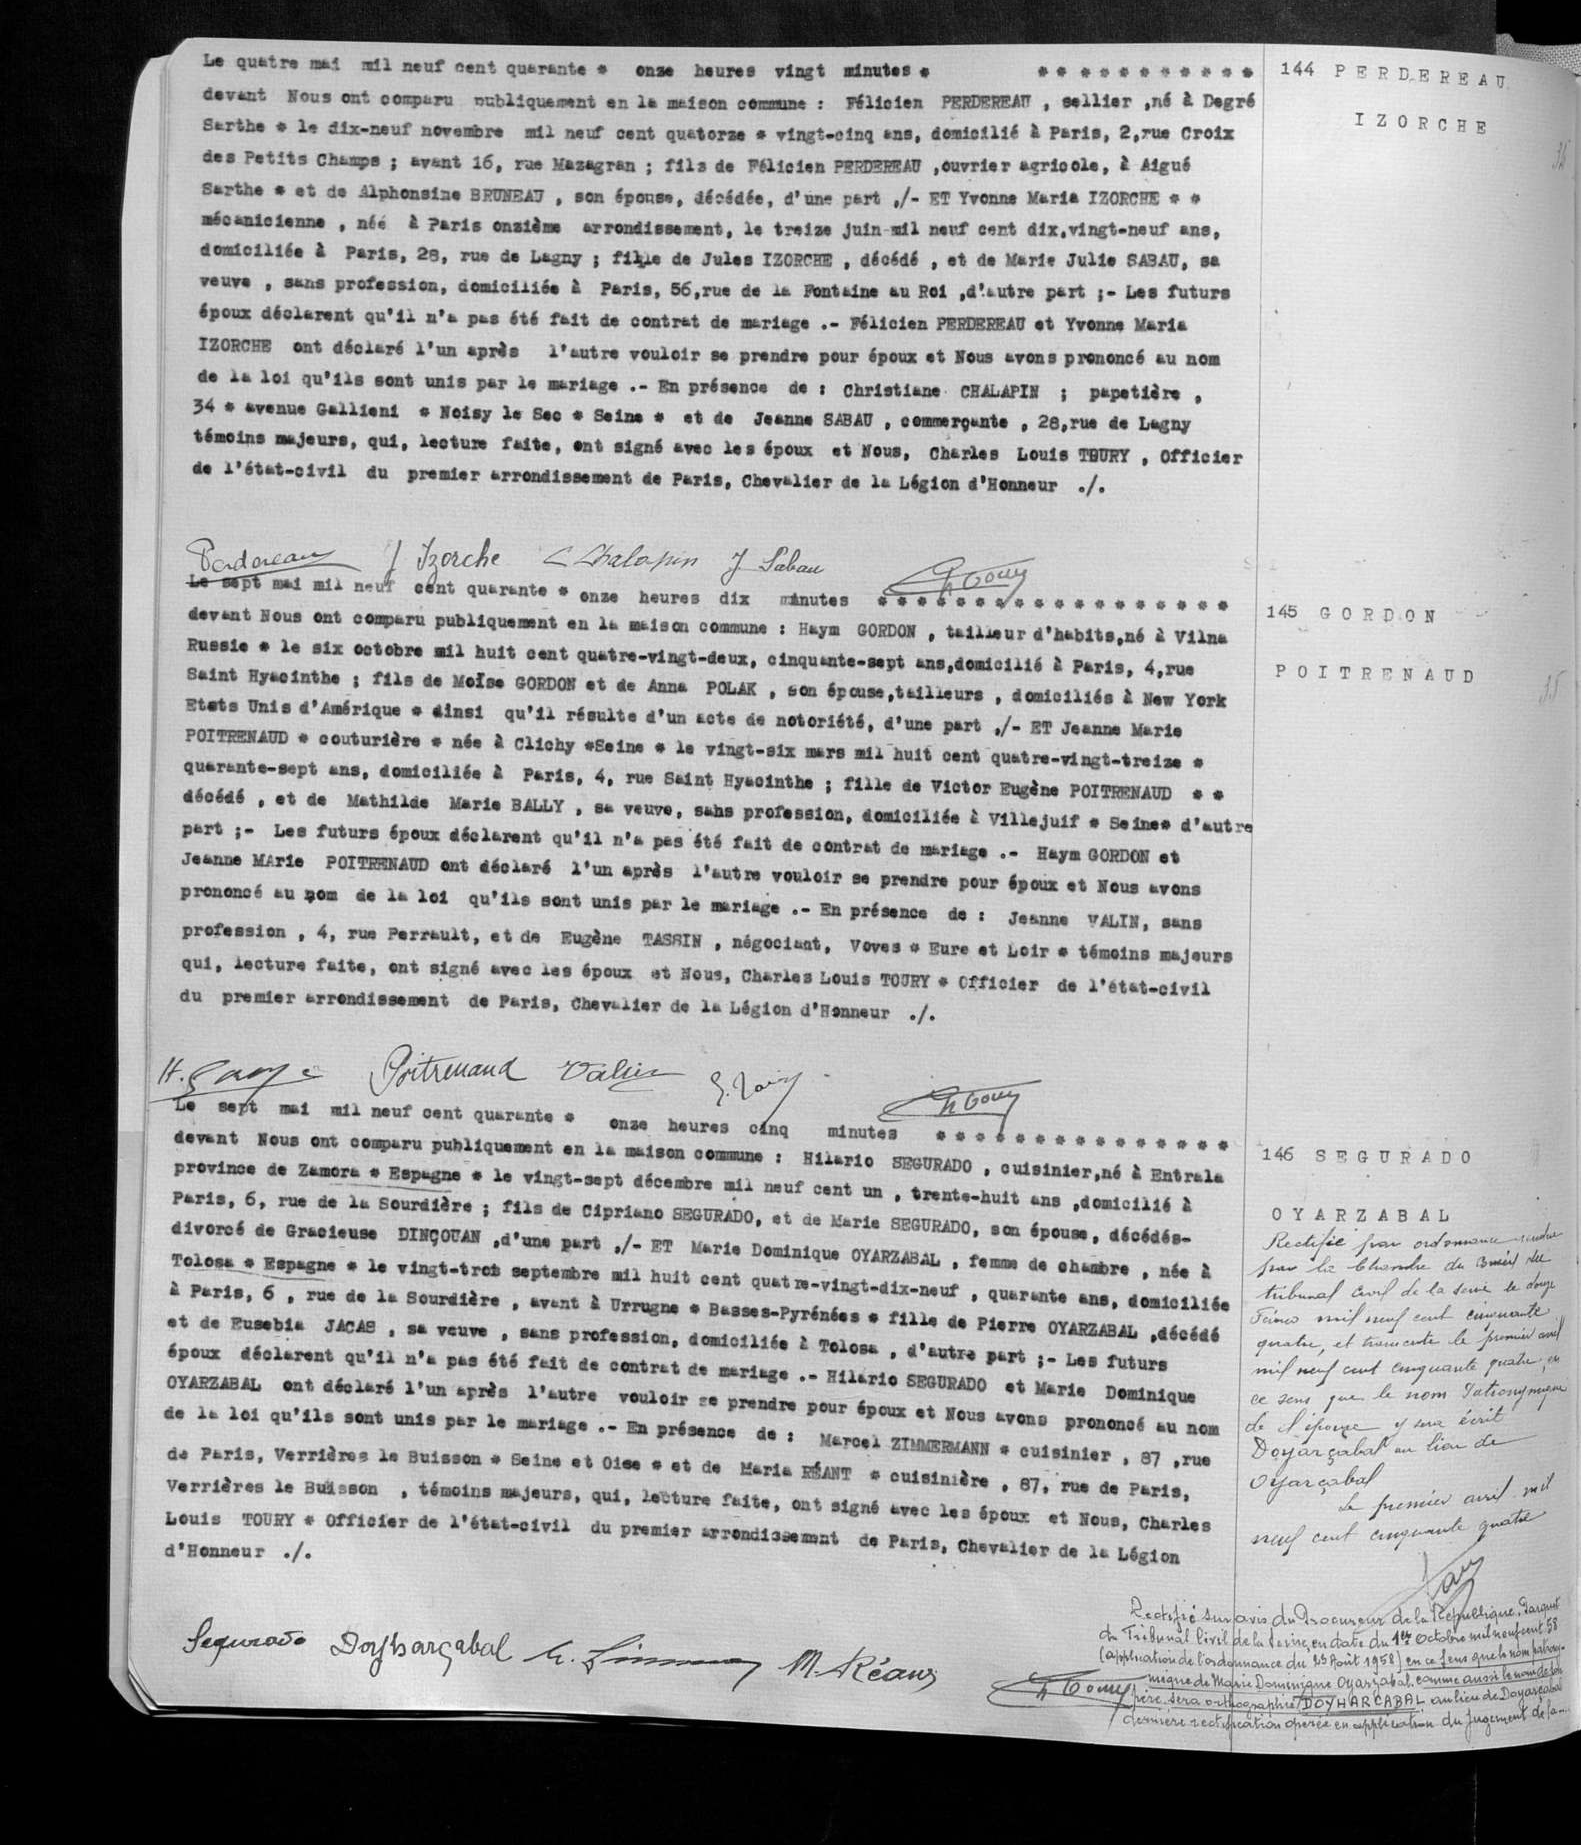Le quatre mai mil neuf cent quarante * onze heures vingt minutes ****
devant Nous ont comparu publiquement en la maison commune: Félicien PERDERAU, sellier, né à
Degré Sarthe * le dix-neuf novembre mil neuf cent quatorze * vingt-cinq ans, domicilié à Paris, 2, rue Croix
des Petits Champs; avant 16, rue Mazagran; fils de Félicien PERDEREAU, ouvrier agricole, à Aigué
Sarthe * et de Alphonsine BRUNEAU, son épouse, décédée, d'une part ,/-ET Yvonne Maria IZORCHE **
mécanicienne, née à Paris onzième arrondissement, le treize juin mil neuf cent dix, vingt-neuf ans,
domicilié à Paris, 28, rue de Lagny; fille de Jules IZORGUE, décédé, et de Marie Julie SABAU, sa
veuve, sans profession, domiciliée à Paris, 56, rue de la Fontaine au Roi, d'autre part ;-Les futurs
époux déclarent qu'il n'a pas été fait de contrat de mariage .-Félicien PERDERAU et Yvonne Maria
IZORCHE ont déclaré l'un après l'autre vouloir se prendre pour époux et Nous avons prononcé au nom
de la loi qu'ils sont unis par le mariage .-En présence de: Christiane CHAPALIN; papetière,
34 * avenue Gallieni * Noise le Sec * Seine * et de Jeanne SABAU, commerçante, 28, rue de Lagny
témoins majeurs, qui, lecture faite, ont signé avec les époux et Nous, Charles Louis TOURY, Officier
de l'état-civil du premier arrondissement de Paris, Chevalier de la Légion d'Honneur ./.
Le sept mai mil neuf cent quarante * onze heures dix minute ****
devant Nous ont comparu publiquement en la maison commune: Haym GORDON, tailleur d'habits, né à Vilna
Russie * le six octobre mil huit cent quatre-vingt-deux, cinquante-sept ans, domicilié à Paris, 4, rue
Saint Hyacinthe; fils de Moïse GORDON et de Anna POLAK, son épouse, tailleurs, domiciliés à New York
Etats Unis d'Amérique * dinsi qu'il résulte d'un acte de notiété, d'une part ,/-ET Jeanne Maire
POITRENAUD * couturière * née à Clichy * Seine * le vingt-six mars mil huit cent quatre-vingt-treize *
quarante-sept ans, domiciliée à Pairs, 4, rue Saint Hyacinthe; fille de Victor Eugène POITRENAUX **
décédé, et de Mathilde Marie BALLY, sa veuve, sans profession, domiciliée à Villejuif * Seine * d'autre
part ;-Les futurs époux déclarent qu'il n'a pas été fait de contrat de mariage .-Haym GORDON et
Jeanne Marie POITRENAUD ont déclaré l'un après l'autre vouloir se prendre pour époux et Nous avons
prononcé au nom de la loi qu'ils sont unis par le mariage .-En présence de: Jeanne VALIN, sans
profession, 4, rue Perrault, et de Eugène TASSIN, négociant, Voves * Eure et Loir * témoins majeurs
qui, lecture faite, ont signé avec les époux et Nous, Charles Louis TOURY * Officier de l'état-civil
du premier arrondissement de Pairs, Chevalier de la Légion d'Honneur ./.
Le sept mai mil neuf cent quarante * onze heures cinq minutes ****
devant Nous ont comparu publiquement en la maison commune: Hilario SEGURADO, cuisinier, né à Entrala
province de Zamora * Espagne * le vingt-sept décembre mil neuf cent un, trente-huit ans, domicilié à
Paris, 6, rue de la Soudière; fils de Cipriano SEGURADO, et de Marie SEGURADO, son épouse, décédés-
divorcé de Gracieuse DINCOUAN, d'une part ,/-ET Marie Dominique OYARZABAL, femme de chambre, née à
Tolosa * Espagne * le vingt-trois septembre mil huit cent quatre-vingt-dix-neuf, quarante ans, domiciliée
à Paris, 6, rue de la Sourdière, avant à Urrugne * Basses-Pyrénées * fille de Pierre OYARZABAL, décédé
et de Eusebia JACAS, sa veuve, sans profession, domiciliée à Tolosa, d'autre part ;-Les futurs
époux déclarent qu'il n'a pas été fait de contrat de mariage .-Hilario SEGURADO et Marie Dominique
OYARZABAL ont déclaré l'un après l'autre vouloir se prendre pour époux et Nous avons prononcé au nom
de la loi qu'ils sont unis par le mariage .-En présence de; Marcel ZIMMERMANN * cuisinier, 87, rue
de Paris, Verrières le Buisson * Seine et Oise * et de Maria REANT * cuisinière, 87, rue de Paris,
Verrières le Buisson, témoins majeurs, qui, lecture faite, ont signé avec les époux et Nous, Charles
Louis TOURY * Officier de l'état-civil du premier arrondissement de Paris, Chevalier de la Légion
d'Honneur ./.
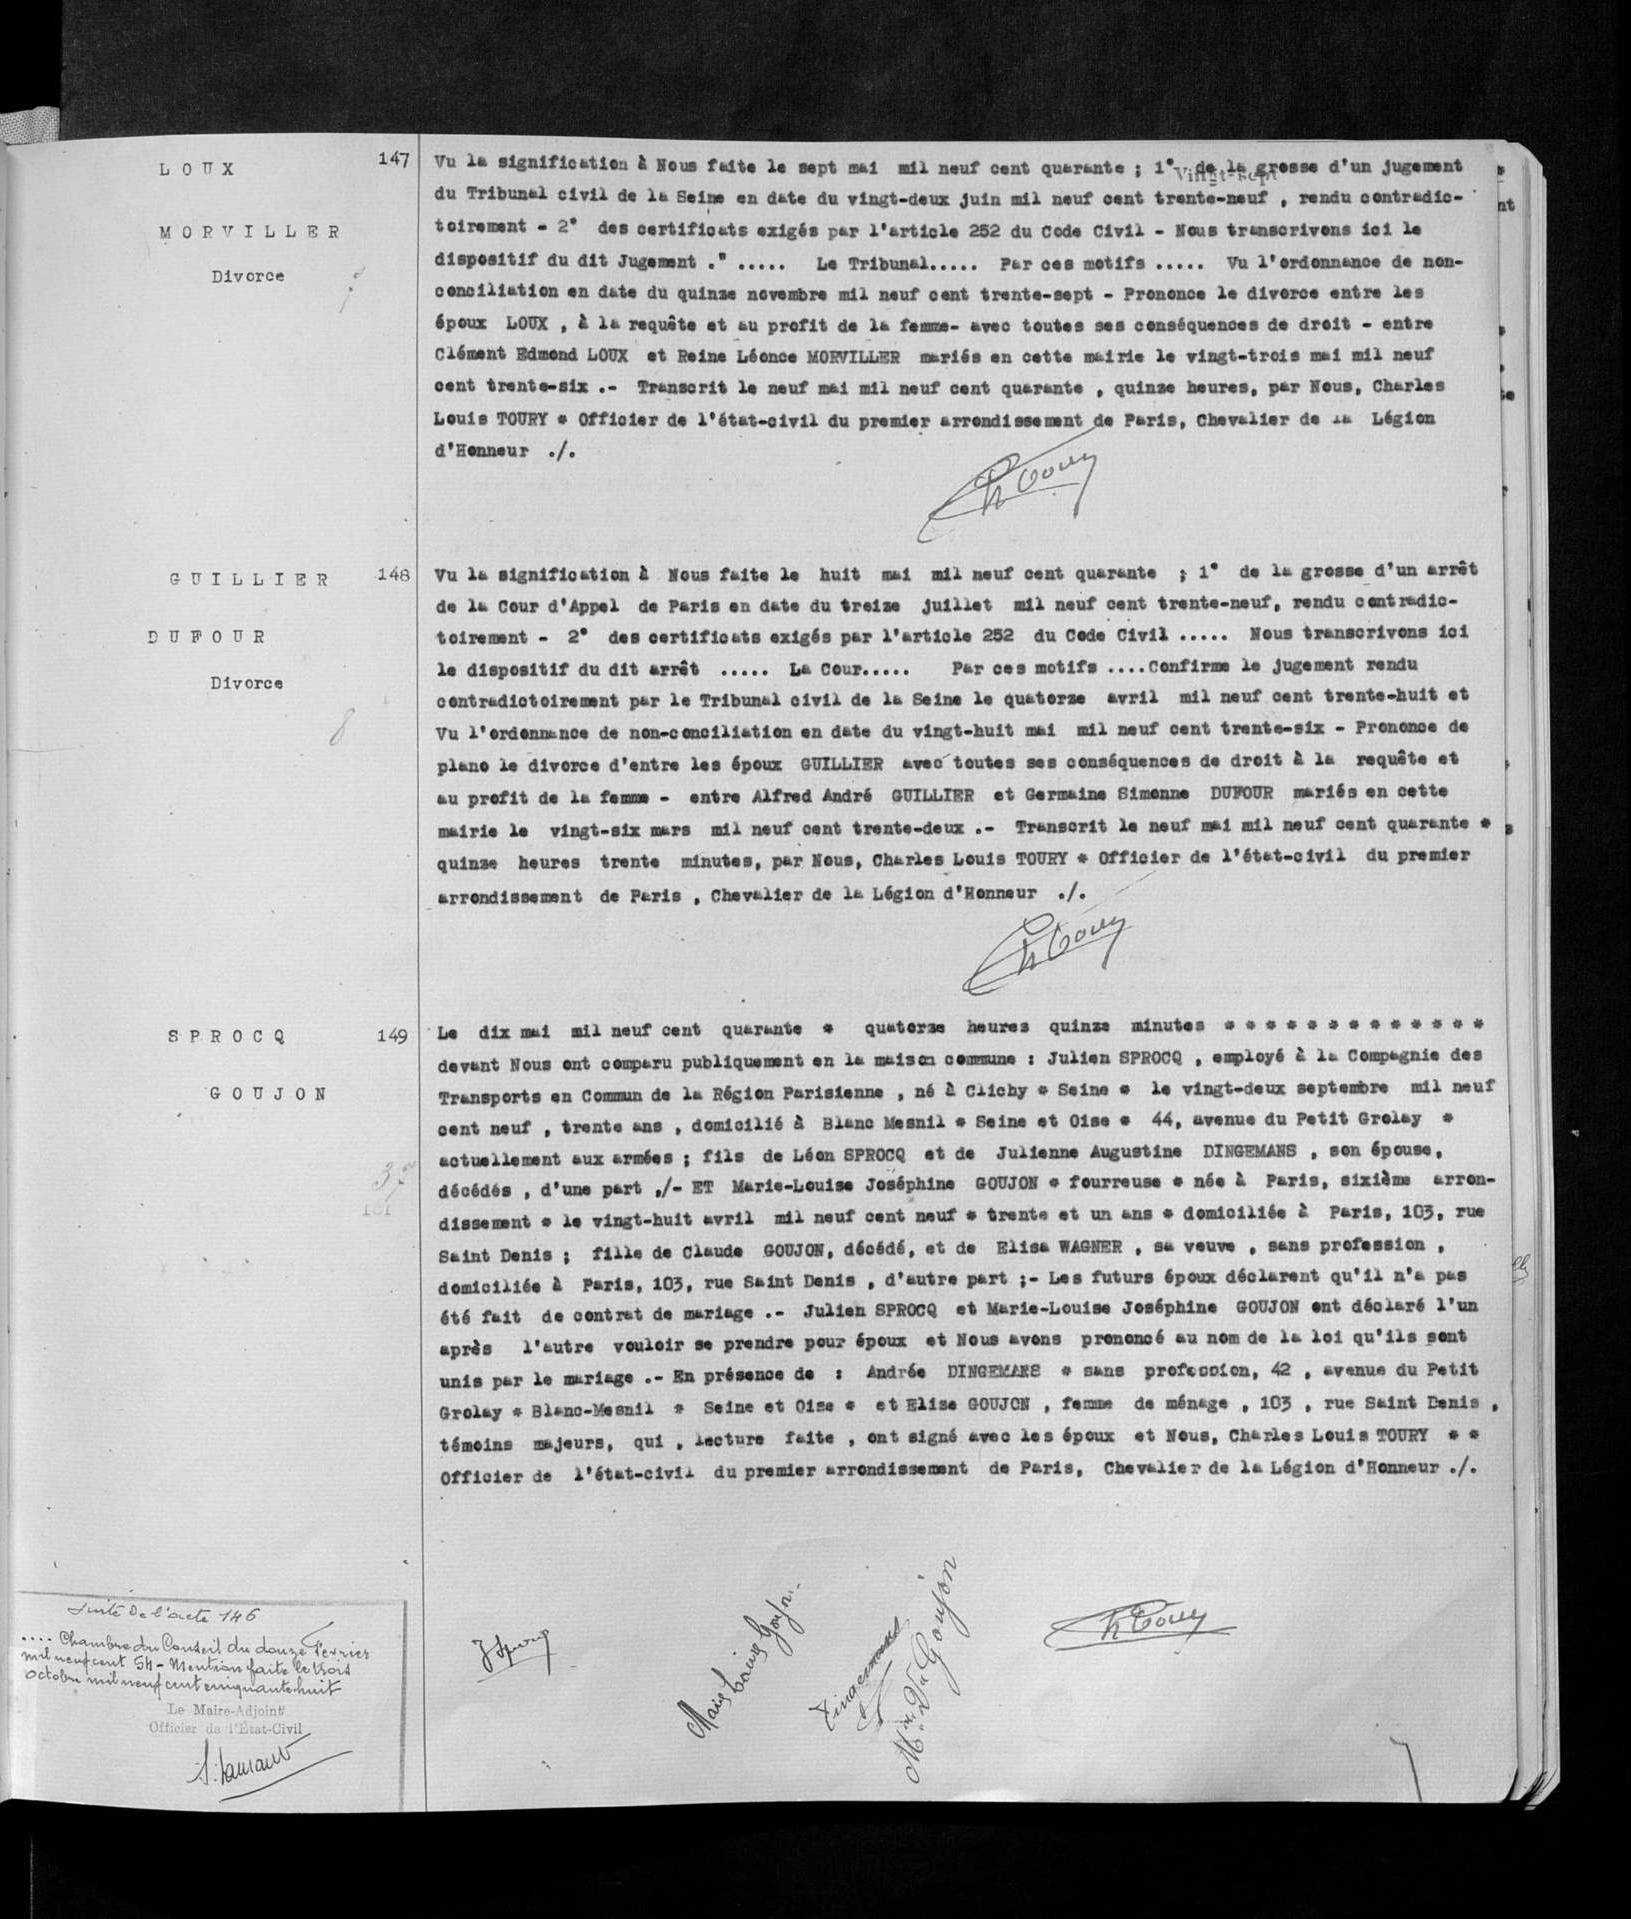Vu la signification à Nous faite le sept mai mil neuf cent quarante; 1° de la grosse d'un jugement
du Tribunal civil de la Seine en date du vingt-deux juin mil neuf cent trente-neuf, rendu contradic-
toirement-2° des certifications exigés par l'article 252 du Code Civil-Nous transcrivons ici le
dispositif du dit Jugement." ..... Le Tribunal..... Par ces motifs..... Vu l'ordonnance de non-
conciliation en date du quinze novembre mil neuf cent trente-sept-Prononce le divorce entre les
époux LOUX, à la requête et au profit de la femme-avec toutes ses conséquences de droit-entre
Clément Edmond LOUX et Reine Léonce MORVIllIER mariés en cette mairie le vingt-trois mai mil neuf
cent trente-six .-Transcrit le neuf mai mil neuf cent quarante, quinze heures, par Nous, Charles
Louis TOURY * Officier de l'état-civil du premier arrondissement de Paris, Chevalier de la Légion
d'Honneur ./.
Vu la signification à Nous faite le huit mai mil neuf cent quarante; 1° de la grosse d'un arrêt
de la Cour d'Appel de Paris en date du treize juillet mil neuf cent trente-neuf, rendu contradic-
toirement-2° des certificats exigés par l'article 252 du Code Civil..... Nous transcrivons ici
le dispositif du dit arrêt..... La Cour..... Par ces motifs.... Confirme le jugement rendu
contradictoirement par le Tribunal civil de la Seine le quatorze avril mil neuf cent trente-huit et
Vu l'ordonnance de non-conciliation en date du vingt-huit mai mil neuf cent trente-six-Prononce de
plano le divorce d'entre les époux GUILLIER avec toutes ses conséquences de droit à la requête
et au profit de la femme-entre Alfred André GUILLIER et Germaine Simonne DUFOUR mariés en cette
mairie le vingt-six mars mil neuf cent trente-deux .-Transcrit le neuf mai mil neuf cent quarante *
quinze heures trente minutes, par Nous, Charles Louis TOURY * Officier de l'état-civil du premier
arrondissement de Paris, Chevalier de la Légion d'Honneur ./.
Le dix mai mil neuf cent quarante * quatorze heures quinze minutes ****
devant Nous ont comparu publiquement en la maison commune: Julien SPROCQ, employé à la Compagnie des
Transports en Commun de la Région Parisienne, né à Clichy * Seine * le vingt-deux septembre mil neuf
cent neuf, trente ans, domicilié à Blanc Mesnil * Seine et Oise * 44, avenue du Petit Grelay *
actuellement aux armées; fils de Léon SPROCQ et de Julienne Augustine DINGEMANS, son épouse,
décédés, d'une part ,/-ET Marie-Louise Joséphine GOUJON * fourreuse * née à Paris, sixième arron-
dissement * le vingt-huit avril mil neuf cent neuf * trente et un ans * domiciliée à Paris, 103, rue
Saint Denis; fille de Claude GOUJON, décédé, et de Elisa WAGNER, sa veuve, sans profession,
domiciliée à Paris, 103, rue Saint Denis, d'autre part ;-Les futurs époux déclarent qu'il n'a pas
été fait de contrat de mariage .-Julien SPROCQ et Marie-Louise Joséphine GOUJON ont déclaré l'un
après l'autre vouloir se prendre pour époux et Nous avons prononcé au nom de la loi qu'ils sont
unis par le mariage .-En présence de: Andrée DINGEMANE * sans profession, 42, avenue du Petit
Grolay * Blanc-Mesnil * Seine et Oise * et Elisa GOUJON, femme de ménage, 103, rue Saint Denis,
témoins majeurs, qui, lecture faite, ont signé avec les époux et Nous, Charles Louis TOURY **
Officier de l'état-civil du premier arrondissement de Paris, Chevalier de la Légion d'Honneur ./.